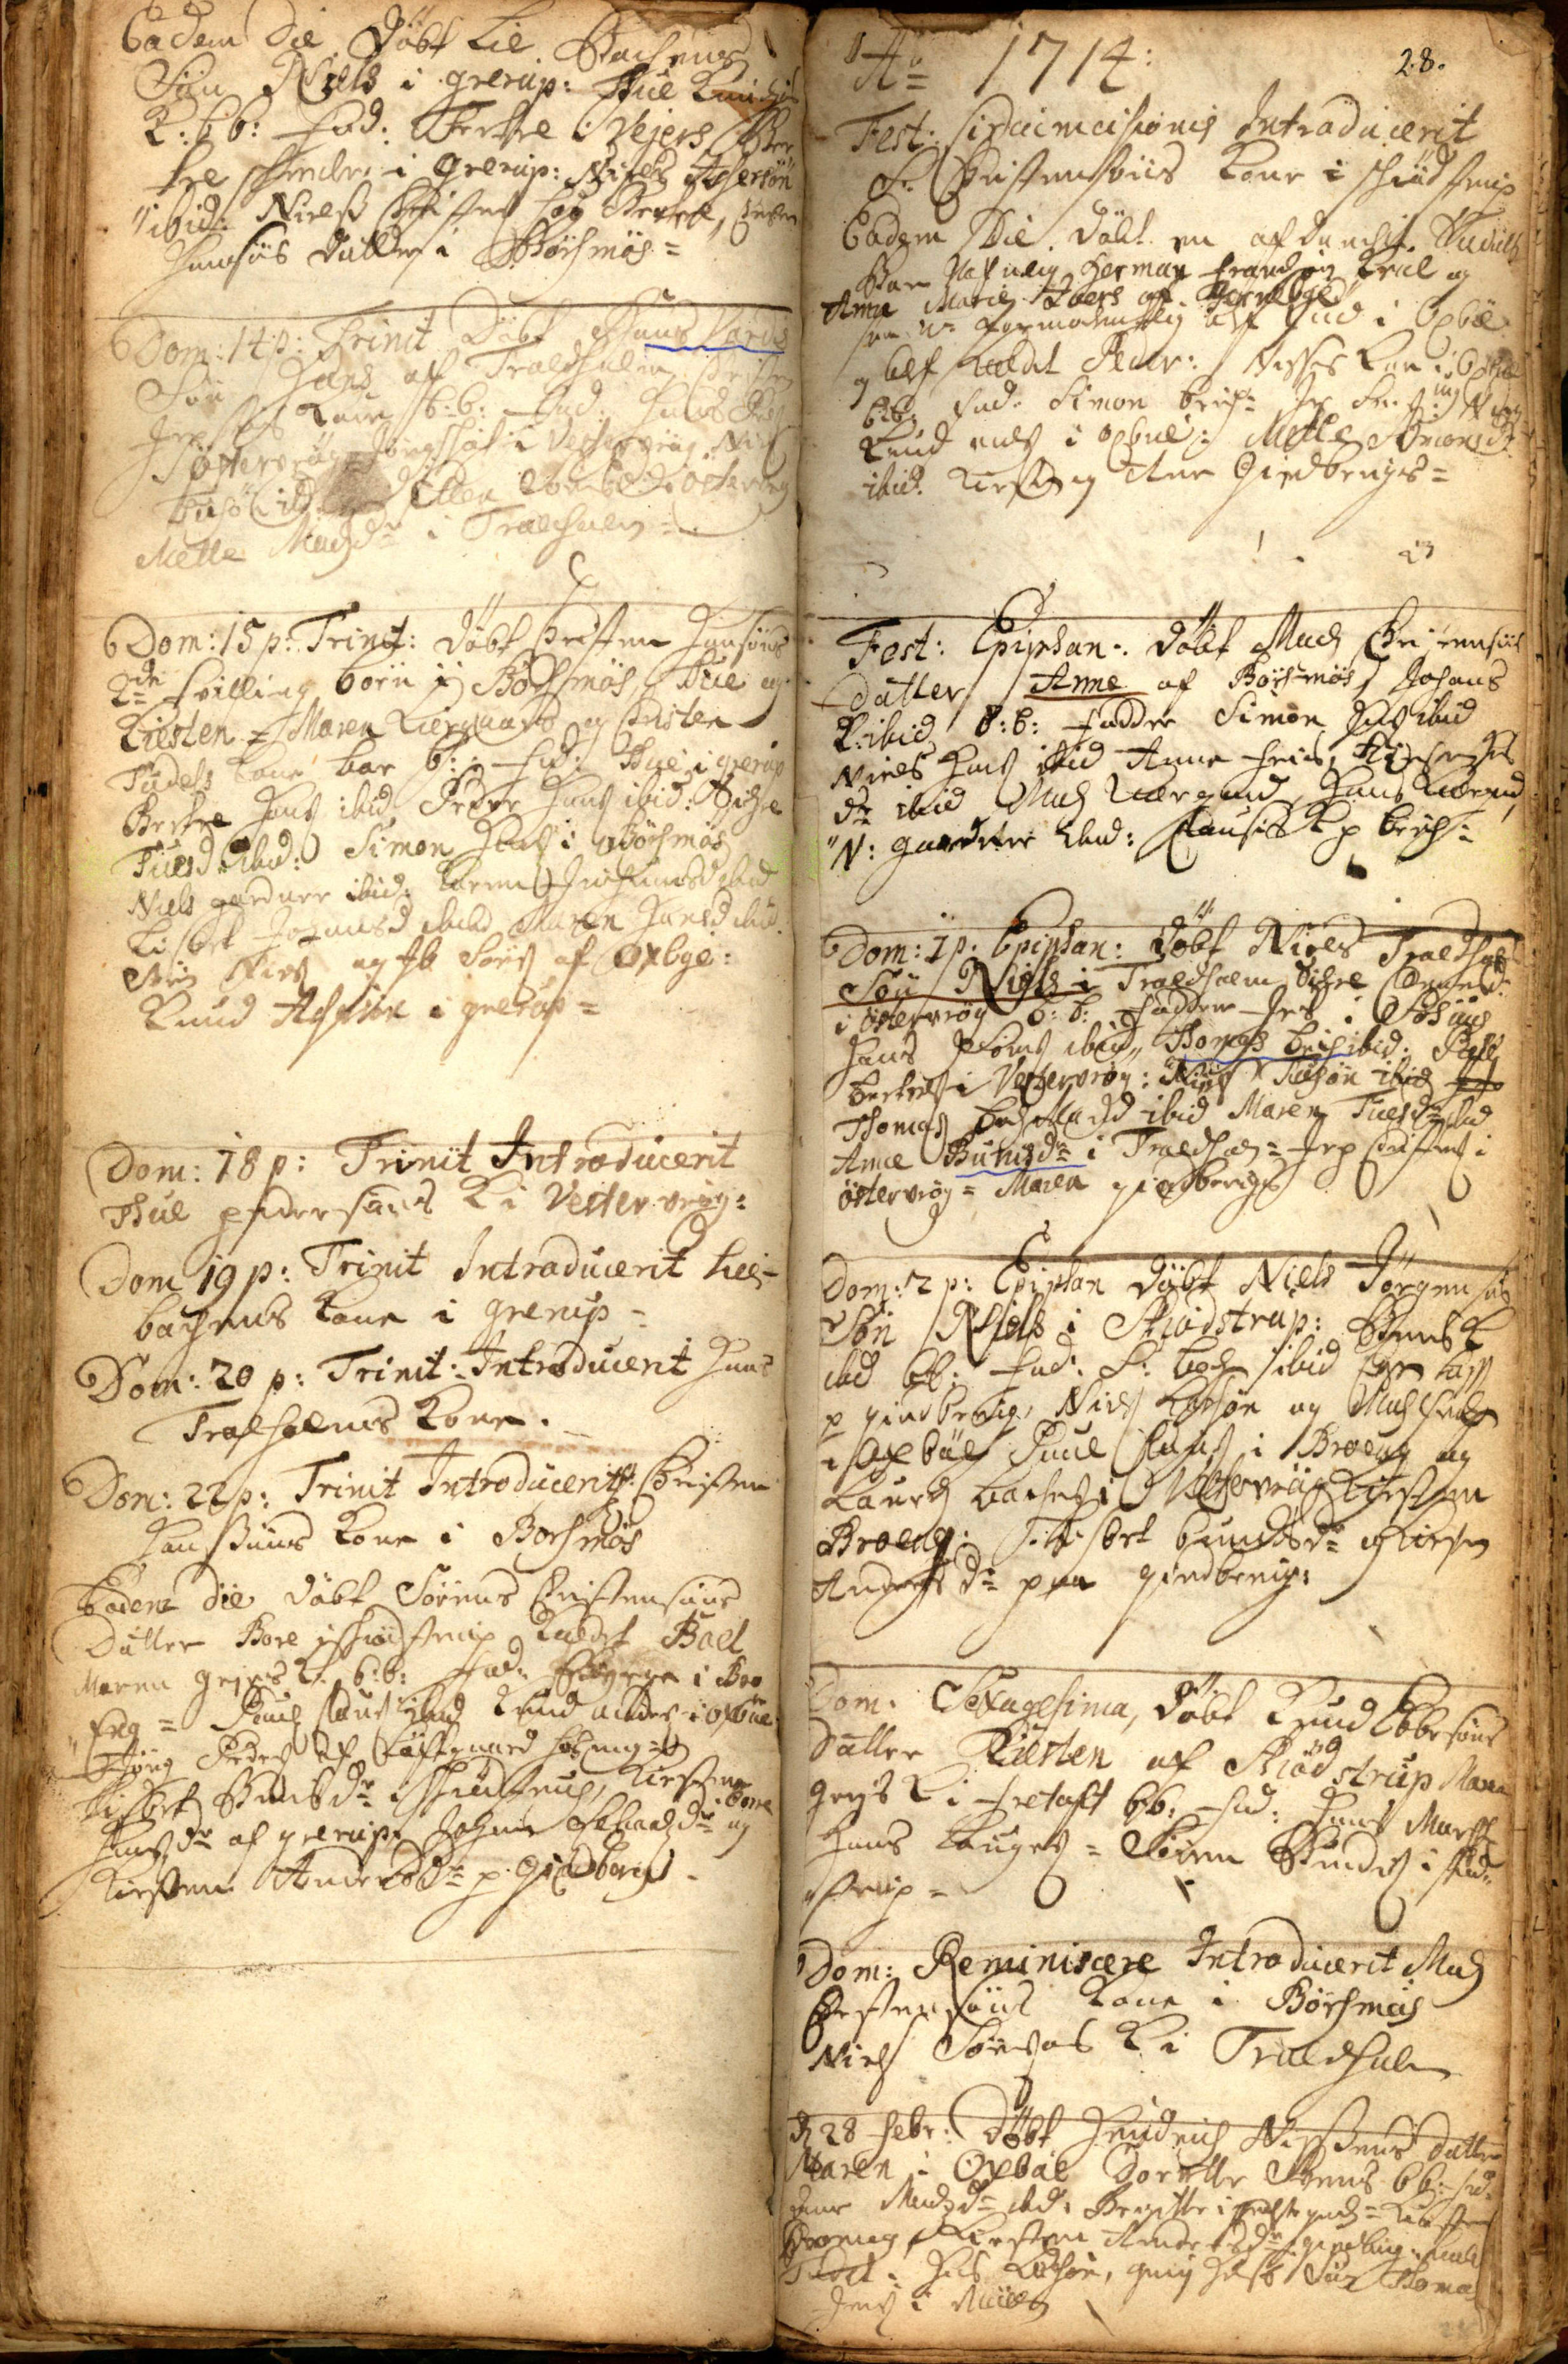Eodem Die Døbt ## Bachens
Søn Niels i Grerup: Thue Knudsens
K: bb: Fad: Terkel i Vejers, Ber¬
tel Schreder i Grerup: Niels Achersøn
ibid: Niels Christens hos Bertel, Christen
Hansøns Datter i Børsmøs=
Dom: 14 p: Trinit Døbt Hans Vardis
Søn Hans af Troldholm, Christen
Jepsøns Kone b:b: Fad: Hans ##
Østervrøg J## i Vestervrøg Ni##
Thusøn ibd ## Ellen Sørensd: i Østervrøg
Mette Madsdr i Troldholm=
Dom: 15 p: Trinit: døbt Christen Hansøns
2de tvilling Børn i Børsmøs, Tue og
Kiesten= Maren Kiergaard og Christen
Tudels Kone bar b: :- Fad: Thue i Grerup
Bertel Hans ibid Peder Hans ibid: Sidzel
Tuesd ibid: Simon Hans i Børsmøs
Niels Gardner ibid: Karen Juchumsd ibid
Lisbet Johansd ibid: Maren Jensd ibid.
Søren Niels og Ib Sørens af Oxbye:
Knud Achersøn i Grerup=
Dom: 18 p: Trinit Introducerit
Thue Pedersøns K i Vestervrøg:
Dom 19 p: Trinit Introducerit Lill-
Bachens Kone i Grerup=
Dom: 20 p: Trinit:  Introducerit Hans
Trolholms Kone.
Dom: 22 p: Trinit Introducerit Christen
Hansøns Kone i Børsmøs
Eodem Die Døbt Sørens Christensøns
Datter Boel i Schiødstrup kaldet Boel
Maren Grjes K: b:b: Fad: Gregers i Bro
eng= Poul Claus ibid Knud ##des i Oxbøl
Jø## Peders af Løftgaard hos mig=
Lisbet Bundisdr i Schiødstrup, Kirsten
##
Hansdr af Grerup: Johanne Seba##dr og
Kirsten Andersdr i Giedberig.
28
28
23
Ao 1714:
Fest: Circumcisionis Introducerit
S: Christensøns Kone i Schiødstrup.
Eodem Die døbt en afdanchit Soldats
Barn Nafnlig Herman Frandzøn Krul og
Anne Marie Ivers af Hennebye,
som er formodentlig ##f fød i Oxbøl
og blef kaldet Peder: Nisses Kone i Oxbøl
b:b: Fad: Simon Brich= Jep ##s: ung N##
Knud niels## i Oxbøl: Mette Sørensdr
ibid: Kirsten og Ane Giedberigs=
Fest: Epiphan: Døbt Madz Christensøns
Datter Anne af Børsmøs, Johans
K: ibid b: b: Fadder Simon Hans ibid
Niels Hans ibid Anne Fris, ##
dr ibid Madz Kiærgaard, Hans Kiærgaard,
N: Gardner ibid: Clausis K p Brich:
Dom: 1 p. Epiphan: Døbt Niels Troldholms
Søn Niels i Troldholm Sidsel Clemensdr
i Østervrøg b: b: Faddere: Jes i Søhuus
Hans Sørens ibid,, Thomas Brich ibid: Palle
Bertels i Vestervrøg: Niels Tuesøn ibid ##
Thomas BodzMandd ibid Maren Tuesdr ibid
Anne Bunnisdr i Troldhol= Jep Christens i
Østervrøg= Maren Giedberigs
Dom:2 p: Epiphan Døbt Niels Jørgensøns
Søn Niels i Skiødstrup: Bunnis## K
ibid bb: Fad: S: Bods ibid, ## Lass
p Giedberig, Niels Lassøn og Madz ##
i Oxbøl, Poul Claus i Broeng og
Lauridz Bachens i Vestervrøg Kirsten
Broeng: Lisbet Bundisdr og Kirsten
Andersdr paa Giedberig:
Dom: Sexagesima Døbt Knud Ebbesøns
Datter Kiesten af Skiødstrup Maren
Grejs K i Fretoft bb: Fad: Hans Marsch##
Hans Lauges= Søren Bundis i Skiød¬
strup=
Dom: Reminiscere Introducerit Madz
Christensøns Kone i Børsmøs
Niels Sørens K i Troldholm
D 28 Febr: Døbt Hendrich Nissens Datter
Maren i Oxbøl Dorrette S##s bb: Fad:
Anne Madzdr ibid, Bergitte i Præstegaard= Kirsten
Broeng, Kirsten Andersdr Giedberig:  Lee##
Tudel##: Jes Lassøn, Gunj## H## Søn Thomas
Jens i ##
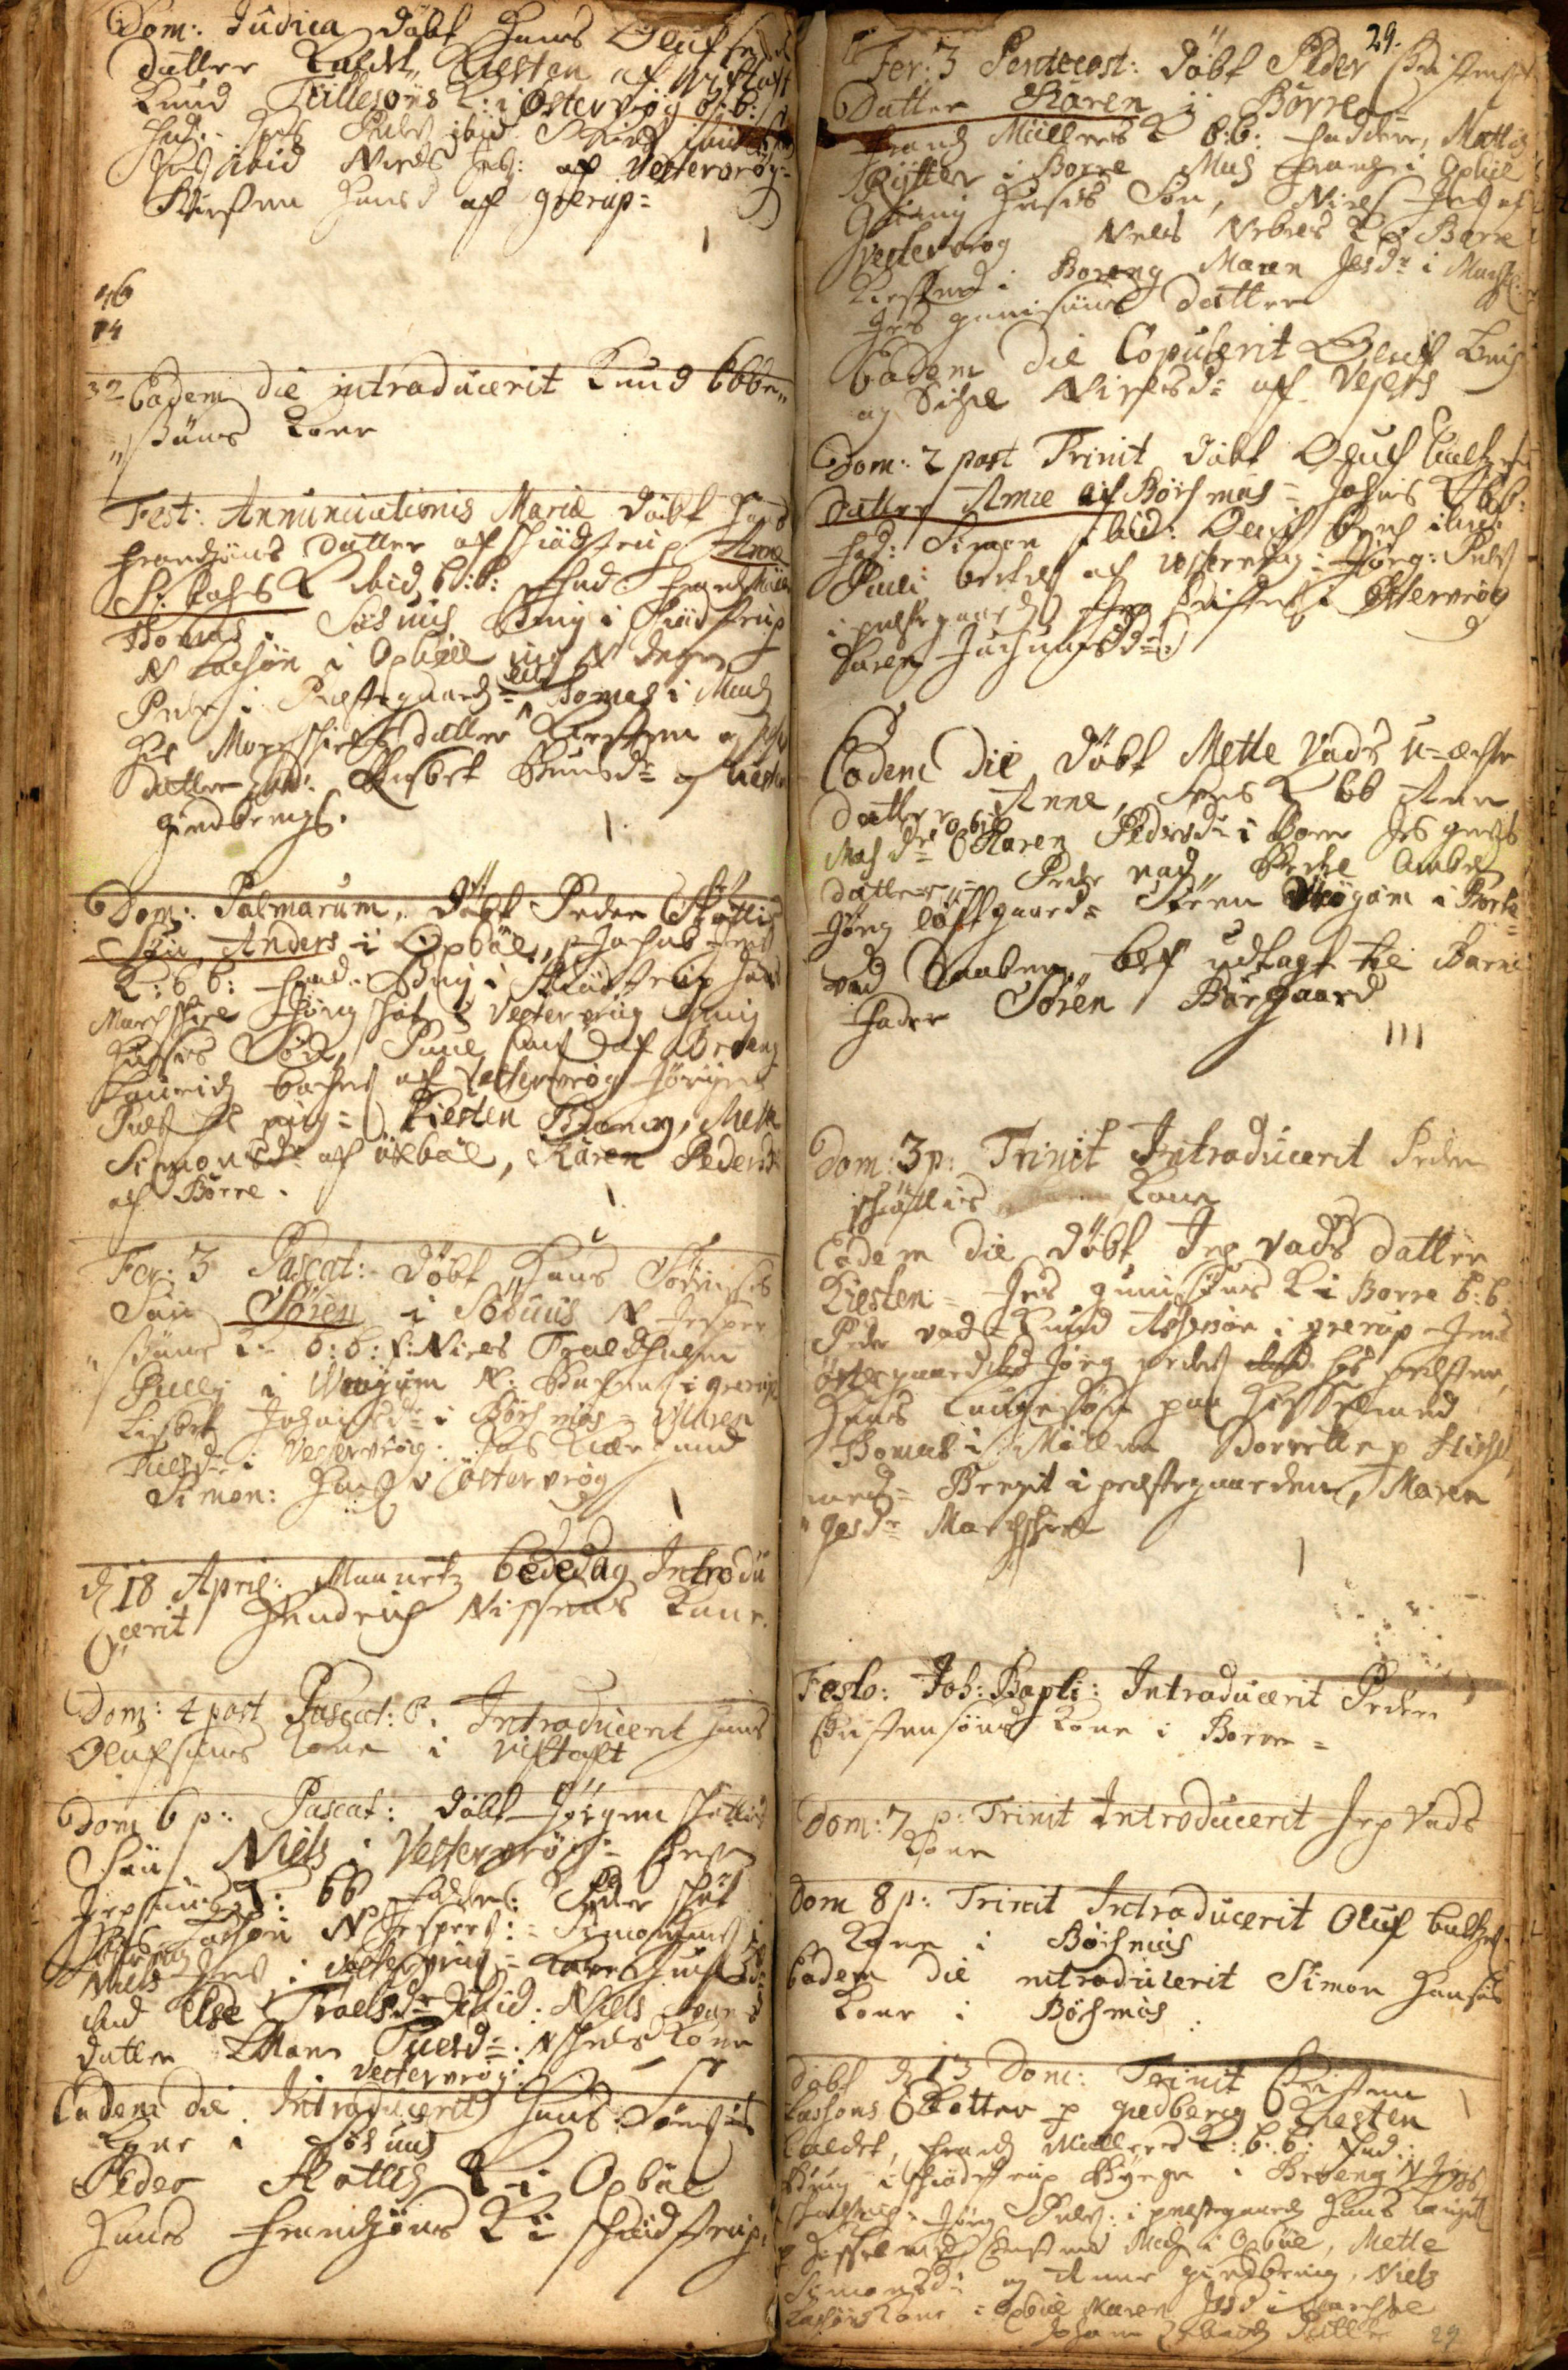Dom: Judica Døbt Hans Olufses
Datter kaldet,, Kiesten af Viftoft,
Knud Tullesøns K: i Østervrøg b: b:
Fad. Hans Peders ibid S## ##
## ibid Niels ##: af Vestervrøg.
Kirsten Hansd af Grerup=
46
74##
32 Eodem Die Introducerit Knud Ebbe¬
søns Kone
Fest: Annunciationis Mariæ Døbt Hans
Frandzøns Datter af Schiødstrup Anne
S: Bahs## K ibid b: b: Fad: Frandz Møller
Thomas i Søhuus, Bunij i Skiødstrup
N Lassøn i Oxbøl ung N Degn
lil##
Per i Præstegaard Thomas i M##
H##s Marchschiels Datter Kirsten og ##
Datter ibid: Lisbet Bundsdr og Kirsten
Giedberigs.
Dom: Palmarum. Døbt Peder Skøttis
Søn Anders i Oxbøl, Jachob Jens
K: b b: Fad: Bunj i Skiødstrup Hans
Marchschiel Jørg Schøt i Vestervrøg Gunj
Hansens Søn, Poul Claus## af Broeng,
Lauridz Bachens af Vestervrøg Jørgen
Tuls hos ## Kiesten Broeng, Mette
Simonsdr af Oxbøl, Karen Pedersd
af Borre.
Fer: 3 Pascat: Døbt Hans Sørenses##
Søn Søren i Søhuus, N Jesper
søns K: b: b: F: Niels Troldholm,
Pallj i Vrøgum N. Bachens i Grerup,
Lisbet Johansdr i Børsmøs= Maren
Tulsdr i Vestervrøg: Hans Kiærgaard,
Simon: Hans i Østervrøg
D 18 Apri:l Maanetz Bededag Introdu¬
cerit Hendrich Nissens Kone.
Dom: 4 post Pascat: ## Introducerit Hans
Oluføns Kone i Viftoft
Dom 6 p: Pascat: Døbt Jørgen Schøttis
Søn Niels i Vestervrøg= Christen
Jepsøns K: bb Fadder: Peder Schøt,
Hans Lassøn N Jespers: = Simon Hans i
Børsmos##
Niels Jens i Vestervrøg= Karen Gundisd
ibid Else Troelsdr ibid: Niels ##
Datter, ## Tuesdr= N ##s Kone
Vestervrøg:
Eodem Die Introducerit Hans Sørensøns
Kone i Søhuus
Peder Skøttis K i Oxbøl
Hans Frandzøns K i Schiødstrup:
29
29
Fer: 3 Pentecost: Døbt Peder Christens
Datter Karen i Borre=
Frandz Møllers K b:b: Fadder, Mathis
Rytter i Borre, Mads ## i Oxbøl,
Gunnj Hansis Søn, Niels Jen##s af
Vestervrøg Niels Nebels K i Borre
Kirsten i Broeng Maren Jesdr i Machschiel##.
Jes Gunnisøns Datter
Eodem Die Copulerit Oluf Brich
og Sidzel Nielsdr af Vejers
Dom: 2 post Trinit Døbt Oluf Baltzers
Datter Anne af Børsmos= Johans K bb:
Fad: Simon ibid: Oluf Brich ibid.
Palli: Bertels af Vestervrøg= Jørg: Peders##
i Præstegaard, Jep Christens i Østervrøg
Karen Jochumsdr
Eodem Die Døbt Mette Vads u-ægte
Datter Anne, ##s K bb, Anne
ibid
Madsdr Karen Pederdr i Borre Jes Guns##
Datter,,= Peder Vad,, Bertel ##
Jørg Løftgaard= Søren Vrøgum i ##=
ved Daaben blef udlagt til Barne
fader Søren Børgaard
Dom: 3 p: Trinit Introducerit Peder
Schøttis Kone
Eodem Die Døbt Jep Vads Datter
Kiesten= Jes Gunisøns K i Borre b: b:
Peder Vad= Knud Assersøn i Grerup Jens
Østergaard ibid Jørg Peders ibid hos Præsten
Hans Laugesøn paa Hisselmed,
Thomas i Møllen, Dorrette p Hissel¬
med= Bergit i Præstegaarden, Maren
Jesdr Marchschiel
Festo: Joh: Bapti: Introducerit Peder
Christensøns Kone i Borre=
Dom: 7 p: Trint Introducerit Jep Vads
Kone
Dom 8 p: Trinit Introducerit Oluf Baltzers
Kone i Børsmøs
Eodem Die introducerit Simon Hansøns
Kone i Børsmøs:
Døbt d 13 Dom: Trinit Christen
Lassøns Datter p Giedberig Kiesten
kaldet, Frandz Møllers K: b: b: Fad.:
Bunj i Schiødstrup Byrge i Broeng, N J##s
Schiødstrup: Jørgen Peders i Præstegaard Hans Lauges
p Hesselmed, Christen Mads i Oxbøl, Mette
Simonsdr og Anne Giedberig, Niels
Lassøns Kone i Oxbøl, Karen Jesd i March##
Johanne Sebæs## datter